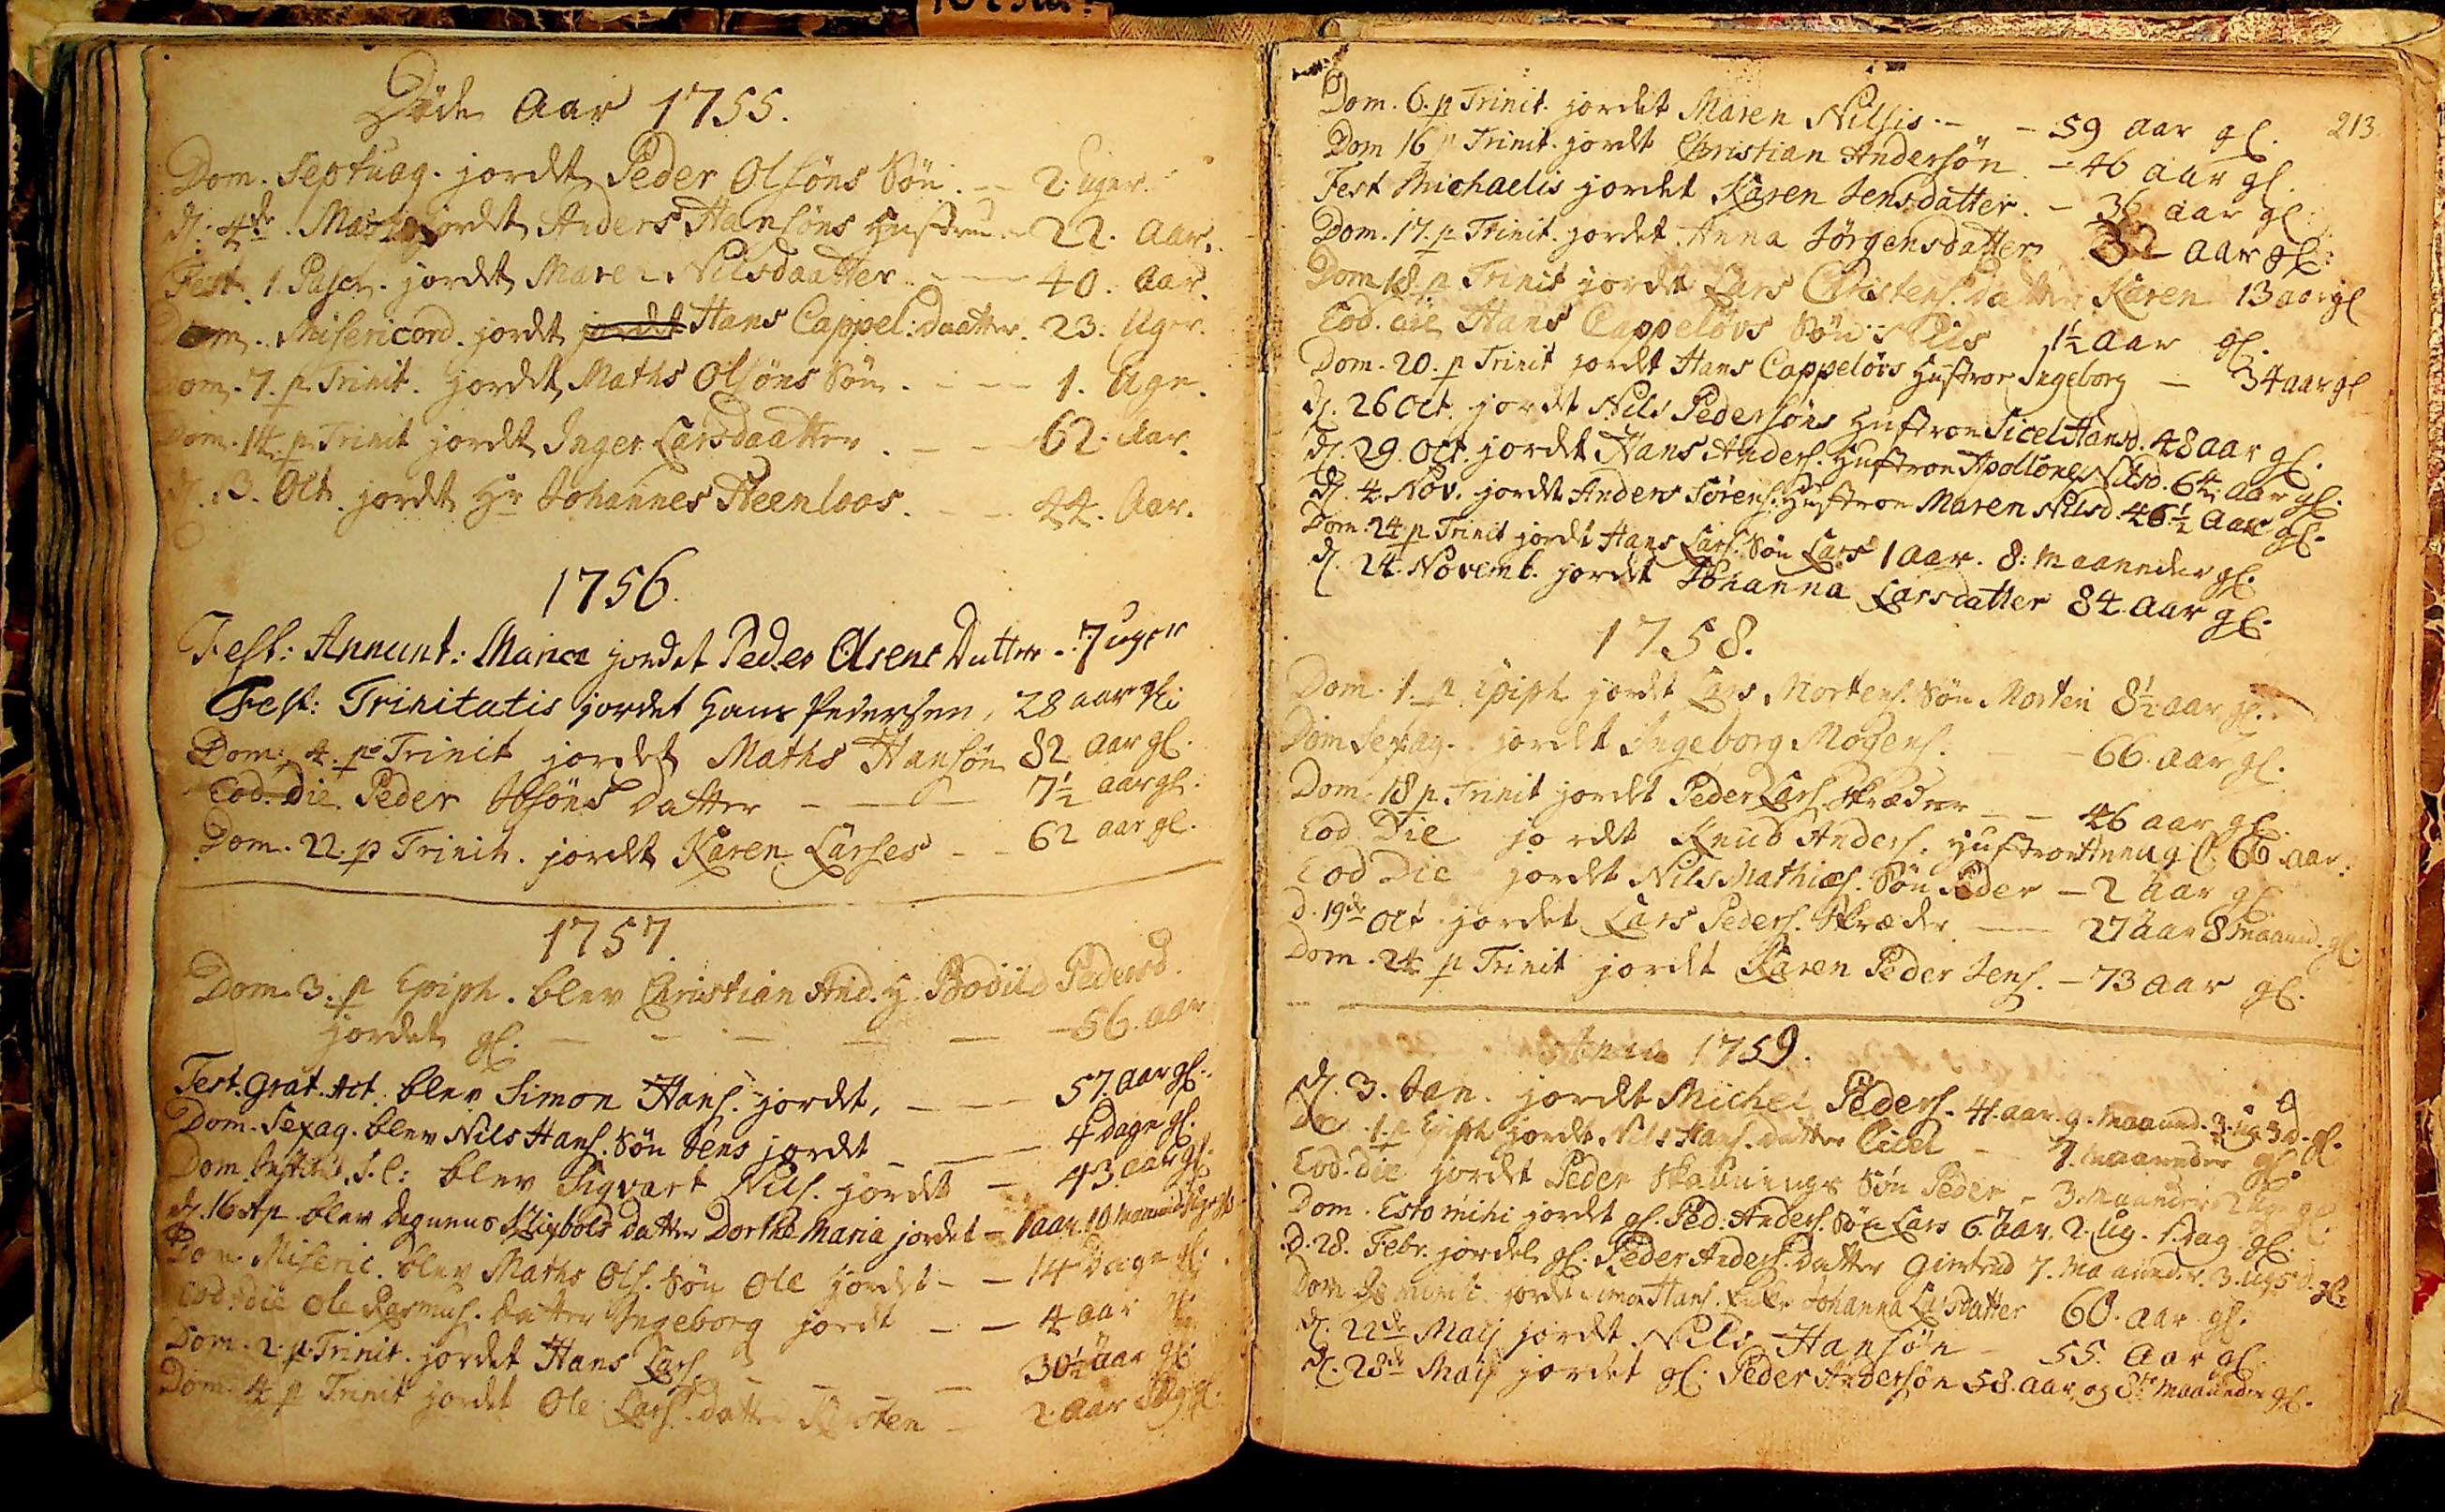Døde Aar 1755.
Dom. Septuag. jordt Peder Olsøns Søn. _ _ 2 Uger.
d: 4de Marts jordt Anders Hansøns Hustrue _ 22. Aar.
Fest 1. Pasch. jordt Maren Nilsdaatter _ _ _ 40. Aar.
Dom. Misericord. jordt jordet Hans Cappel: Daatter. 23 Uger.
Dom. 7. p Trinit. jordet Maths Olsøns Søn. _ _ _ 1. Uge
Dom. 14. p Trinit jordt Inger Larsdaatter. _ _ 62. Aar.
d. 13. Oct. jordt Hr Johannes Steenloos. _ _ 44. Aar.
1756.
Fest: Annunt: Mariæ jordet Peder Olsens Datter. 7 Uger
Fest: Trinitatis jordet Hans Pedersen 28 Aar gl.
Dom: 4. p. Trinit jordet Maths Hansøn 82 Aar gl.
Eod. die. Peder Ibsøns Datter _ _ _ 7½ Aar gl.
Dom. 22. p Trinit. jordt Karen Larses _ _ 62 Aar gl.
1757.
Dom. 3. p Epiph. blev Christian And. H. Bodild Pedersd.
jordet gl. _ _ _ _ _ _ _ _ _ _ _ _ _ _ _  56 Aar.  
Fest. Grat. Act. blev Simon Hans. jordt, _ _ _ _ 57 Aar gl.
Dom. Sexag. blev Nils Hans. Søn Jens jordt _ _ _ 4 Dage gl.
Dom Justi##, J.C: blev Sigvart Nils. jordt _ 43 Aar gl.
d. 16. Ap. blev Degnens Klixbols datter Dorthe Maria jordet _ 1 Aar. 10 Maaned 1 Uge gl.
Dom. Miseric. blev Maths Ols. Søn Ole jordet _ _ 14 Dage gl.
Eod: die Ole Rasmus. Datter Ingeborg jordt _ _ 4 Aar gl.
Dom. 2. p. Trinit. jordet Hans Lars. _ _ _ _ _ _ 30½ Aar gl:
Dom. 4 p Trinit jordet Ole Lars. datter Kirsten _ 2 Aar 8 Ug gl.
213.
Dom. 6. p Trinit. jordet Maren Nilsis. __ 59 Aar gl.
Dom. 16 p Trinit. jordt Christian Andersøn. __ 46 Aar gl.
Fest Michaelis jordet Karen Jensdatter __ 36 Aar gl:
Dom. 17. p Trinit. jordet Anna Jørgensdatter 32 Aar gl:
Dom 18. p Trinit jordet Lars Christens. Datter Karen 13 Aar gl:
Eod. die Hans Cappeløvs Søn Nils 1½ Aar gl.
Dom 20. p Trinit jordt Hans Cappløvs Hustroe Ingeborg __ 34 Aar gl
d. 26 Oct. jordt Nils Pedersøns Hustroe Sicel Hansd. 48 Aar gl. 
d. 29 Oct. jordt Hans Anders. Hustroe Apollone Nilsd. 64 Aar gl.
d. 4. Nov. jordt Anders Sørens. Hustroe Maren Nilsd. 46½ Aar gl.
Dom. 24 p Trinit jordt Hans Lars. Søn Lars 1 Aar. 8. Maaneder gl.
d. 24 Novemb. jordt Johanna Larsdatter 84 Aar gl.
1758. 
Dom. 1. p Epiph jordt Lars Mortens. Søn Morten 8½ Aar gl.
Dom Sexag. jordt Ingeborg Mogens. __ __ 66 Aar gl.
Dom. 18 p . Trinit jordt Peder Lars Skræder __ __ 46 Aar gl.
Eod. Die jordt Knud Anders. Hustroe Anna gl 66 Aar. 
Eod die jordt Nils Mathiæs. Søn Peder ____ 2 Aar gl
d. 19de Oct. jordet Lars Peders. Skræder ____ 27 Aar 8 Maaned. gl.
Dom 24 p Trinit jordt Karen Peder Jens. __ 73 Aar gl.
Anno 1759.
d. 3. Jan. jordt Michel Peders. 41 aar. 9 maaned. 3 uger 3 d. gl.
Dom. 1. p Epiph jordt Nils Hans. Datter Sicel ____ 7 maaneder gl.
Eod. die jordt Peder Skannings Søn Peder. - 3 maaneder 2 Ug gl
Dom. Esto mihi jordet gl. Ped. Anders. Søn Lars 6 aar. 2. Ug. 1 Dag gl
D. 28. Febr. jordet gl. Peder Anders. Datter Giertrud 7 maaneder 3 Ug. 5d. gl.
Dom Reminsc. jordt Simon Hans. Enke Johanna Larsdatter 60 Aar gl.
d. 22de Maij jordt Nils Hansøn __ 55 Aar gl.
d. 28de Maij jordet gl. Peder Andersøn 58 Aar og 8te maaneder gl.
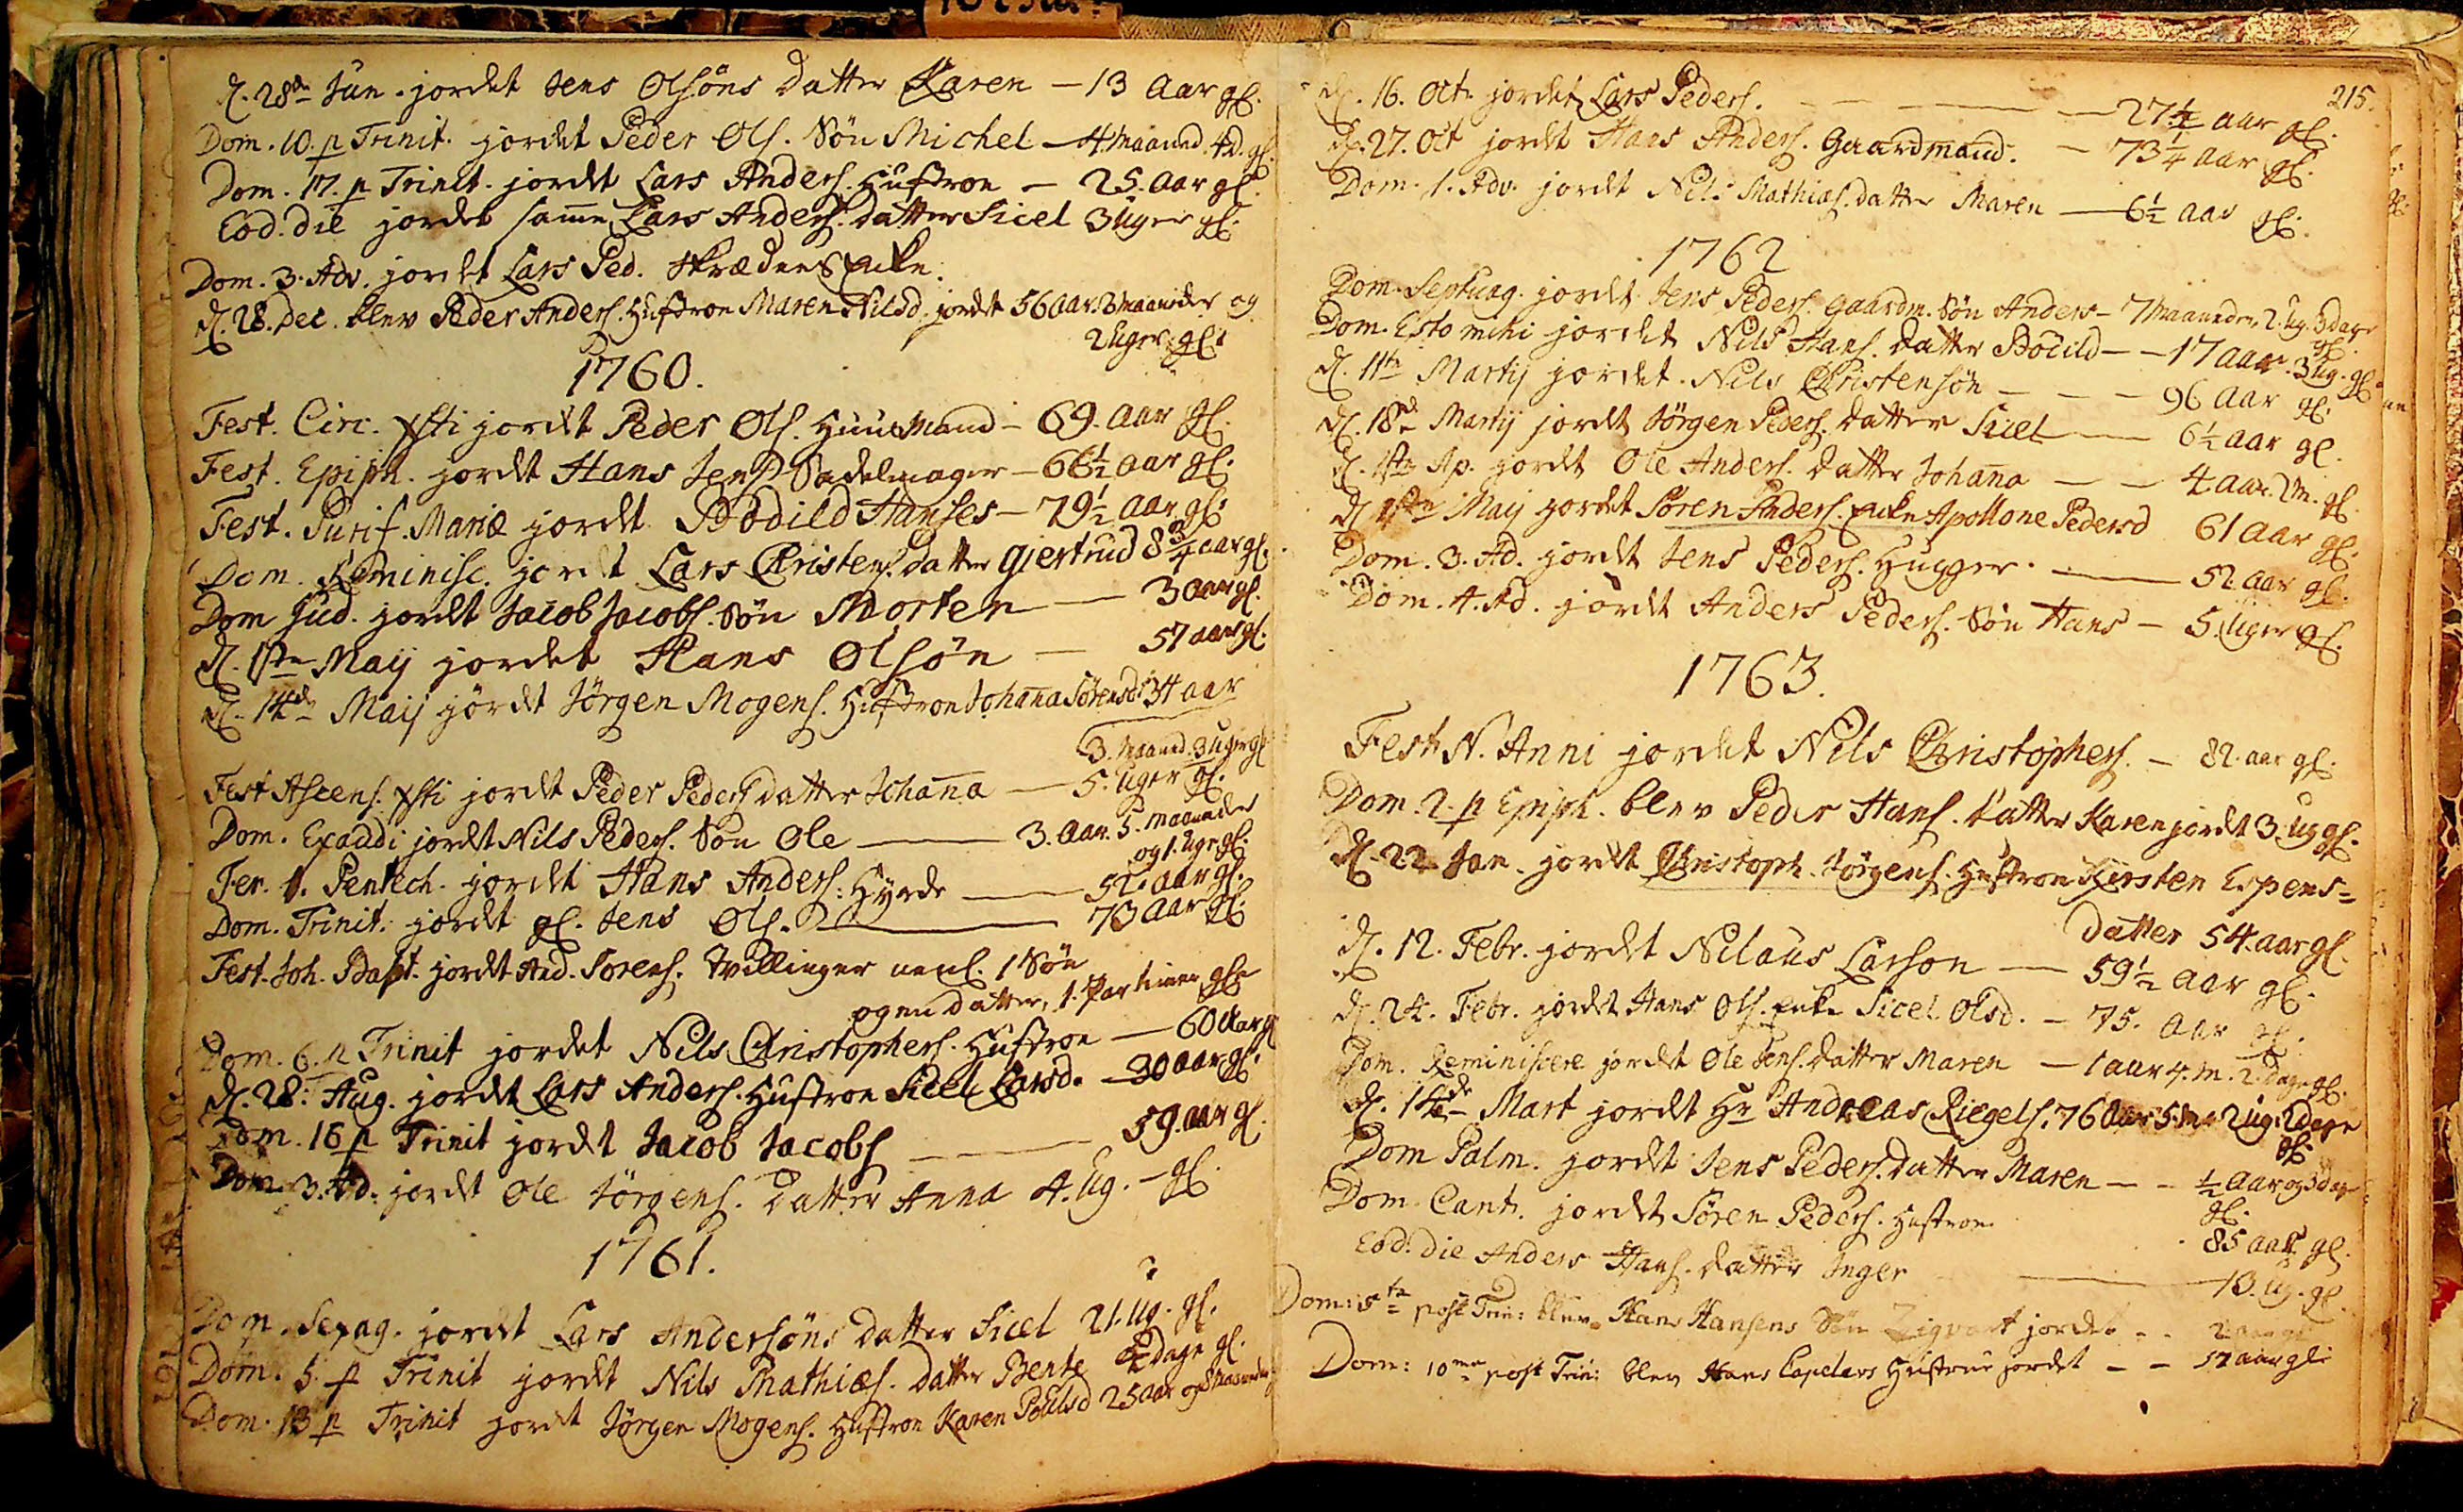d. 28de Jun. jordet Jens Olsøns Datter Karen __ 13 Aar gl.
Dom. 10. p Trinit. jordet Peder Ols. Søn Michel __ 4 maaneder. 4 d. gl.
Dom. 17. p Trinit. jordet Lars Anders. Hustroe __ 25 Aar gl.
Eod. die jordet samme Lars Anders. datter Sicel 3 Uger gl.
Dom. 3. Adv. jordet Lars Ped. Skræders Enke.
d. 28. Dec blev Peder Anders. Hustroe Maren Nilsd. jordet 56 Aar. 3 Maaneder og
2 Uger. gl.
1760.
Fest. Circ. Xsti jordet Peder Ols. Huusmand __  69. Aar gl.
Fest. Epiph. jordet Hans Jens Sadelmager _ 66½ Aar gl.
Fest. Purif. Mariæ jordt Bodild Hanses __ 79½ Aar gl.
Dom Reminisc jordt Lars Christens. Datter Giertrud 8¾ Aar gl.
Dom Jud. jordet Jacob Jacobs. Søn Morten ____  3 Aar gl.
d. 1ste Maij jordet Hans Olsøn ___ 57 Aar gl.
d. 14de Maij jordt Jørgen Mogens. Hustroe Johanna Sørensd: 34 Aar
3. Maaned. 3 Uger gl
Fest Ascens. Xsti jordt Peder Peders Datter Johanna __ 5. Uger gl.
Dom. Exaudi jordt Nils Peders. Søn Ole _____ 3. Aar 5. Maaneder
og 1. Uge gl.
Fer. 1. Pentech. jordt Hans Anders: Hyrde _________ 52. Aar gl.
Dom. Trinit: jordt gl. Jens Ols. ___________________ 73 Aar gl.
Fest. Joh. Bapt. jordt And. Sorens. tvillinger neml. 1 Søn
og en datter.  1. Par timer gl.
Dom. 6. p Trinit jordet Nils Christophers. Hustroe ____ 60 Aar gl.
d. 28: Aug. jordet Lars Anders. Hustroe Sicel Larsd. __ 30 Aar gl.
Dom. 16 p Trinit jordt Jacob Jacobs __________________ 59. Aar gl.
Dom. 3 Ad. jordt Ole Jørgens Datter Anna 4. Ug. -- gl.
1761.
Dom. Sexag. jordet Lars Andersøns Datter Sicel 21. Ug. gl.
Dom. 5. p Trinit jordet Nils Mathiæs. Datter Berte 4 Dage gl.
Dom. 13 p Trinit jordt Jørgen Mogens. Hustroe Karen Poulsd 25 Aar og 8 Maaneder
215.
d. 16. Oct. jordet Lars Peders. ___ ___ ___ ____ 27½ Aar gl.
d. 27. Oct jordt Hans Anders. Gaardmand. __ 73¼ Aar gl.
Dom. 1. Adv. jordt Nils Mathiæs. datter Maren _____ 6½ Aar gl.
1762
Dom.Septuag. jordt Jens Peders. Gaardm. Søn Anders _ 7 Maaneder, 2. Ug. 3 Dage
gl.
Dom. Esto mihi jordet Nils Hans. Datter Bodild __ __ 17 Aar. 3 Ug. gl.
d. 11te Martii jordet Nils Christensøn __ __ __ 96 Aar gl.
d. 18de Martii jordt Jørgen Peders. Datter Sicel ________ 6½ Aar gl.
d. 1ste Ap. jordt Ole Anders. Datter Johanna __ __ 4 Aar. 2 M. gl.
d. 1ste Maij jordet Søren Anders. Enke Apollone Peders 61 Aar gl.
Dom. 3. Ad. jordt Jens Peders. Hugger. _________ 52. Aar gl.
Dom. 4. Ad. jordt Anders Peders. Søn Hans __ 5 Uger gl.
1763.
Fest N. Anni jordet Nils Christophers. ___ 82. Aar gl.
Dom. 2. p Epiph. blev Peder Hans. Datter Karen jordet 3 Ug gl.
d. 22 Jan. jordt Christoph. Jørgens. Hustroe Kirsten Espens¬
datter 54. Aar gl.
d. 12. Febr. jordt Nilaus Larson ____ 59½ Aar gl.
d. 24. Febr. jordet Hans Ols. Enke Sicel Olsd. ____ 75 Aar gl.
Dom. Reminiscere jordet Ole Jens. Datter Maren __ 1 Aaar 4. M. 2. Dage gl.
d. 14de Mart jordt Hr Andreas Riegels. 76 Aar 5 M. 2 Ug: 2 Dage
gl.
Dom Palm. jordet Jens Peders. Datter Maren __ __ __ ½ Aar og 3 dage
gl.
Dom. Cant. jordet Søren Peders. Hustroe 85 Aar gl.
Eod.die Anders Hans. datter Inger _________________ 13 Ug. gl.
Dom: 5ta post Trin: blev Hans Hansens Søn Zigvart jordet __ __ 2 Aar gl.
Dom: 10ma post Trin: Hans Capelevs Hustrue jordet __ __ 57 Aar gl.